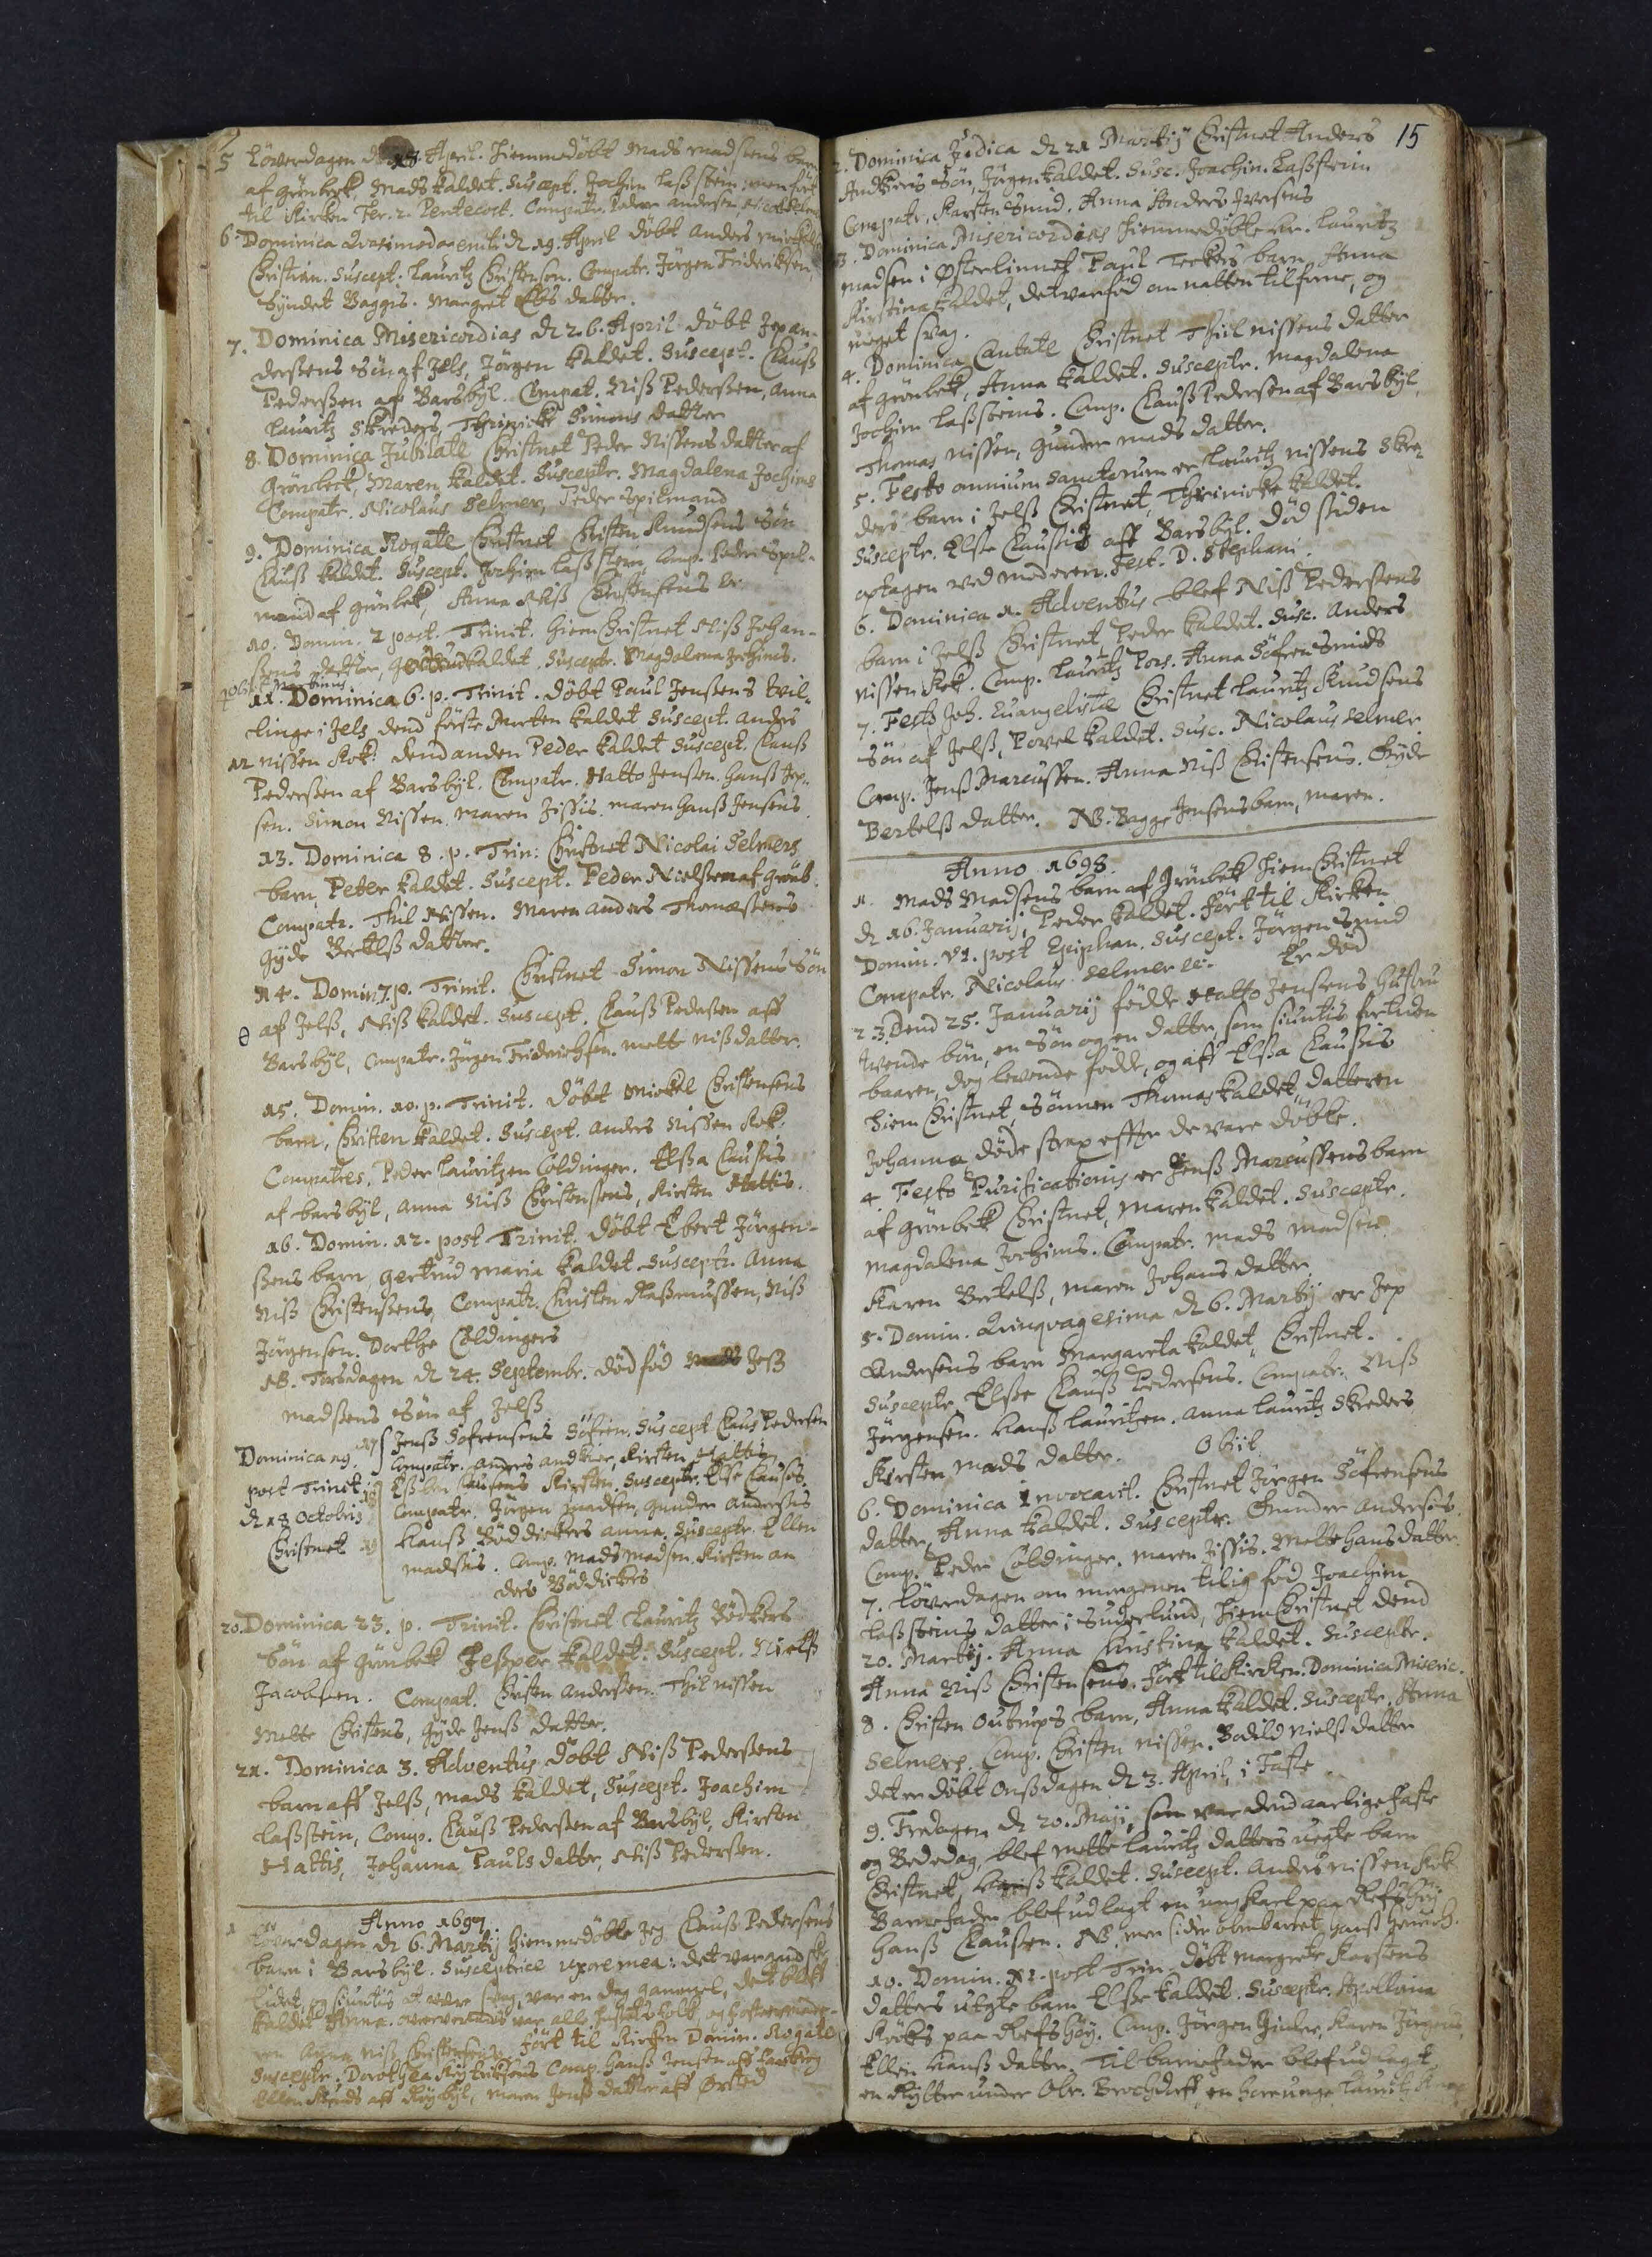5. Løverdagen d 18 April. Hiemmedøbt Mads Madsens barn
af Grønbeck, Mads kaldet. Suscept. Jochim Lasstein, men ført
til Kirken Fer. 2. Pentecost. Compatr. Peder Andersen, Nicol## Selm##
6. Dominica Qvasimodogeniti d 19. April døbt Anders Michelsens
Christian. Suscept. Lauritz Christensen. Compatr. Jørgen Friderichsen,
Syndet Baggis. Margret Ebs datter.
7. Dominica Misericordias d 26. April døbt Jep An¬
dersens Søn af Jels, Jørgen kaldet. Suscept. Claus
Pedersen af Barsbyl. Compat. Niss Pedersen, Anna
Lauritz Skreders, Thrinicke Simons datter.
8. Dominica Jubilate Christnet Peder Nissens datter af
Grønbeck, Maren kaldet. Susceptr. Magdalena Jochims
Compatr. Nicolaus Selmer, Peder Spilmand
9. Dominica Rogate Christnet Christen Knudsens Søn
Claus kaldet. Suscept. Jochim Lasstein Comp. Peder Spil¬
mand af Grønbek, Anna Niss Christensens
10. Domin. 2 post Trinit. Hiem Christnet Niss Johan¬
sens datter, Gertrud kaldet. Suscept. Magdalena Jochims.
obiit mortinus##
11. Dominica 6. p. Trinit. døbt Paul Jensens tvil¬
linge i Jels dend første Morten kaldet. Suscept. Anders
12. Nissen Kok: Dend anden Peder kaldet. Suscept. Claus
Pedersen af Barsbyl. Compatr. Hatto Jensen. Hans Jep¬
sen. Simon Nissen. Maren Jissis. Maren Hans Jensens.
13. Dominica 8. p. Trin. Christnet Nicolai Selmers
barn Peder kaldet. Suscept. Peder Nielsen af Grønb.
Compatr. Thil Nissen. Maren Anders Thomæsens
Gyde Bertelss datter.
14. Domin. 7. p. Trinit. Christnet Simon Nissens Søn
af Jels, Niss kaldet . Suscept. Claus Pedersen aff
Barsbyl, Compatr. Jørgen Fredrichsen. Mette Nissdatter.
15. Domin. 10. p. Trinit. døbt Mickel Christensens
barn, Christen kaldet. Suscept. Anders Nissen Kok.
Commatres. Peder Lauritzen Coldinger. Elssa Clausis
af Barsbyl, Anna Niss Christensens, Kirsten Hattis.
16. Domin. 12. post Trinit. Døbt Ebert Jørgen¬
ssens barn, Gertrud Maria kaldet. Suscept. Anna
Niss Christensens. Compatr. Christen Rasmussen, Niss
Jørgensen. Dorthe Coldingers
NB. Torsdagen d 24. septembr. dødfød ## Jess
Madsens Søn af Jelss
Dominica 19.
post Trinit,
d 18 Octobris
Christnet
17 Jens Søfrensens Søfrin. Suscept Claus Pedersen
Compatr. Anders Andkier, Kirsten Hattis
18 Essben Clausens Kirsten. Susceptr. Else Clausis.
Compatr. Jürgen Madsen. Gunder Andersis
19 Hans Bøddickers Anna. Susceptr. Ellen
Madsis. Comp. Mads Madsen. Kirsten An
ders Bøddickers
20. Dominica 23. p. Trinit. Christnet Lauritz Bødkers
Søn af Grønbek Jesper kaldet. Suscept. Niels
Jacobsen. Compat. Christen Andersen. Thil Nissen.
Mette Christens. Gyde Jenss datter.
21. Dominica 3. Adventus døbt Niss Pedersens
barn aff Jels, Mads kaldet. Suscept. Joachim
Lasstein, Comp. Claus Pedersen af Barsbyl, Kirsten
Hattis, Johanna Pauls datter, Niss Pedersen.
Anno 1697.
1 Løverdagen d 6 Martij hiemmedøbte jeg Claus Pedersens
barn i Barsbyl. Susceptrice uxoremea: det var
lidet, og siuntis at være svag, var en dag gammel, det bleff
kaldet Anna. overværendes var alle husets folk og fostemoder
var Anna Niss Christensens. Ført til kirken Domin. Rogate
Susceptr. Dorthea Key Eriksens Comp. Hans Jensen aff
Ellen Svends aff Roybyl, Maren Jenss datter aff Ørsted
15
2. Dominica Judica d 21 Martij Christnet Anders
Andkieris Søn, Jørgen kaldet. Susc. Joachim Lasstein
Compatr. Karsten Smid. Anna Anders Iversens
3. Dominica Misericordias hiemmedøbte Hr. Lauritz
Madsen i Østerlinnet Paul Teckers barn, Anna
Kirstina kaldet, det var fød om natten tilforn, og
meget svag.
4. Dominica Cantate Christnet Thiil Nissens datter
af Grønbeck, Anna kaldet. Susceptr. Magdalena
Jochim Lassteins. Comp. Claus Pedersen af Barsbyl,
Thomas Nissen, Gunder Mads datter.
5. Festo Onnium Sanctenum er Lauritz Nissens Skre¬
ders barn i Jels Christnet, Thrinicke kaldet.
Susceptr. Else Clausis aff Barsbyl. Død siden
optagen ved moderen. Fest. D. Stephani.
6. Dominica 1. Adventus blef Niss Pedersens
barn i Jels Christnet Peder kaldet. Susc. Anders
Nissen Kok. Comp. Lauritz Pors. Anna Søfren Smids.
7. Festo Joh. Euangeliste Christnet Lauritz Knudsens
Søn af Jels, Povel kaldet. Susc. Nicolaus Selmer.
Comp. Jens Marcussen. Anna Niss Christensens, Gyde
Bertels datter. NB. Bagge Jensens barn, Maren.
Anno 1698.
1. Mads Madsens barn af Grønbek hiem Christnet
d 16 Januarij, Peder kaldet. Ført til Kirken
Domin. VI. post Epiphan . Suscept. Jørgen Smid
Compatr. Nicolaus Selmer
Er død
2. 3. Dend 25. Januarij fødde Hatto Jensens hustru
tvende børn, en søn og en datter, som siuntis for tiden
baaren, dog levende fødde, og aff Elssa Clausis
hiem Christnet, Sønnen Thomas kaldet, datteren
Johanna døde strax efter de vare døbte.
4. Festo Purificationis er Jens Marcussens barn
af Grønbeck Christnet, Maren kaldet. Susceptr.
Magdalena Jochims. Compatr. Mads Madsen.
Karen Bertelss, Maren Johans datter.
5. Domin. Qvinqvagesima d 6. Martij er Jep
Andersens barn Margareta kaldet, Christnet.
Susceptr Else Claus Pedersens. Compatr. Niss
Jørgensen. Hans Lauritzen. Anna Lauritz Skreders.
Kirsten Mads datter.
Obiit
6. Dominica Invocavit. Christnet Jørgen Søfrensens
datter, Anna kaldet. Susceptr. Gunder Andersis.
Comp. Peder Coldinger. Maren Jissis. Mette Hans datter.
7. Løverdagen om morgenen tilig fød Joachim
Lassteins datter i Suderlund, hiem Christnet dend
20. martij. Anna Kristine kaldet. Susceptr.
Anna Niss Christensens. Ført til Kirken Dominica Miseric.
8. Christen Outrups barn. Anna kaldet. Susceptr. Anna
Selmers. Comp. Christen Nissen. Bodild Niels datter
Det er døbt Onsdagen d 3. April i Faste.
9. Fredagen d 20. Maji, som var dend aarlige faste
og Bededag, blef Mette Lauritz datters uegte barn
Christnet, Hans kaldet. Suscept. Anders Nissen Kok
Barnefader blef udlagt en ungkarl paa Refshøy
Hans Clausen. NB. Men siden obenbarret Hans Henrich.
10. Domin. XI. post Trin. døbt Margrete Karstens
datters uegte barn Else kaldet. Susceptr. Apollina
Køcks paa Refshøy. Comp. Jørgen Hiuler, Karen Jørgens,
Ellen Hans datter. Til Barnefader blef udlagt
en Rytter under Obr. Brockdorff, en horeunge Lauritz